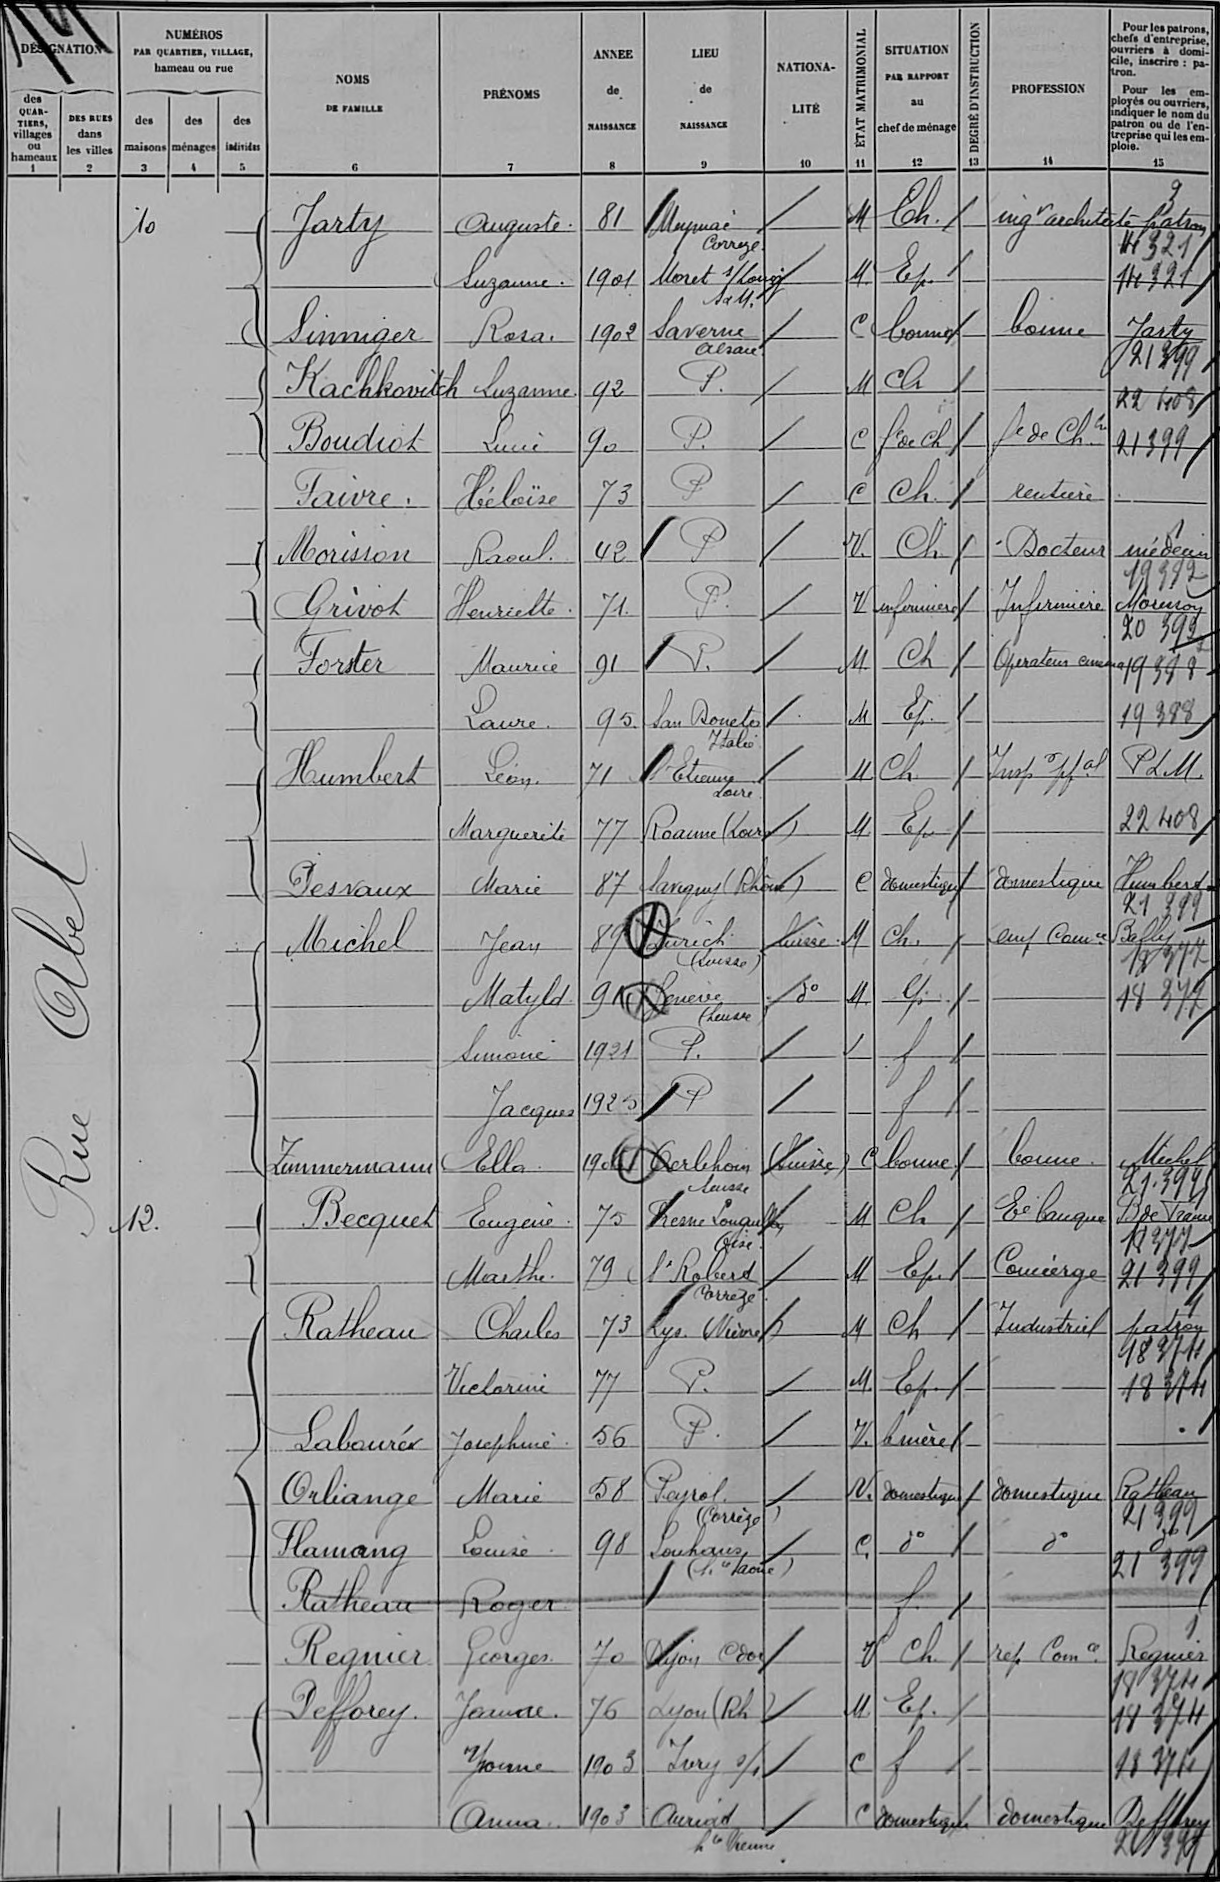Jarty/Auguste/81/meymac!correze/¤/M/Ch /¤/ingr architecte/patron!14321
¤/Suzanne/1901/Moret-Loing!S&M/¤/M/Ep /¤/¤/14321
Sinniger/Rosa/1902/Saverne!Alsace/¤/C/bonne/¤/bonne/Jarty!21399
Kachkovitch/Suzanne/92/P /¤/M/Ch/¤/¤/22408
Boudioh/Lucie/90/P /¤/C/Fe de Ch/¤/Fe de Ch/21399
Faivre/Héloïse/73/P/¤/C/Ch /¤/rentière/¤
Morisson/Raoul/42/P/¤/V /Ch/¤/Docteur/médecin!19382
Grivoh/Henriette/71/P /¤/V/Infirmière/¤/Infirmière/Moruroy !20392
Forster/Maurice/91/P /¤/M/Ch/¤/Opérateur cinema/19388
¤/Laure/95/San Donato!Italie/¤/M/Ep /¤/¤/19388
Humbert/Léon/71/St Etienne!Loire/¤/M/Ch/¤/Insp ppal PdM/¤
¤/Marguerite/77/Roanne (Loire)/¤/M/Ep/¤/¤/22408
Desnaux/Marie/87/Savigny (Rhône)/¤/E/domestique/¤/domestique/Humbert !21399
Michel/Jean/89/Zurich!(Suisse)/Suisse/M/Ch /¤/emp Comce/Bally!18377
¤/Matyld/91/Geneve!(Suisse)/d°/M/Ep /¤/¤/18372
¤/Simone/1921/P /¤/¤/f/¤/¤/¤
¤/Jacques/1925/P/¤/¤/f/¤/¤/¤
Zimmermann/Ella/1904/Oerlikon !Suisse/(Suisse)/C/bonne/¤/bonne/Michel!21399
Becquet/Eugene/75/Fresnes Longuillon !Oise/¤/M/Ch/¤/Eé banque/B de France!18377
¤/Marthe/79/St Robert !Corrèze/¤/M/Ep /¤/Concierge/21399
Ratheau/Charles/73/Lys (Nièvre)/¤/M/Ch/¤/Industriel/patron!18374
¤/Vieloruie/77/P /¤/M /Ep /¤/¤/18374
Labouré/Josephine/56/P /¤/V /b mère/¤/¤/¤
Orliange/Marie/58/Peyrol!(Corrèze)/¤/V /domestique/¤/domestique/Ratbeau!21399
Hamang/Louise/98/Louhans!(hte Saone)/¤/C/d°/¤/d°/d° !21399
Ratheau/Roger/¤/¤/¤/¤/f/¤/¤/¤
Regnier/Georges/70/Dijon Cdor/¤/V/Ch /¤/rep Comce/Regnier!18374
Defforey/Jeanne/76/Lyon (Rh)/¤/M/Ep /¤/¤/18194
¤/Yvonne/1903/Ivry/¤/C/f/¤/¤/18374
¤/Anna/1903/Auriat ! Hte Vienne/¤/C/domestique/¤/domestique/Defforey!21399
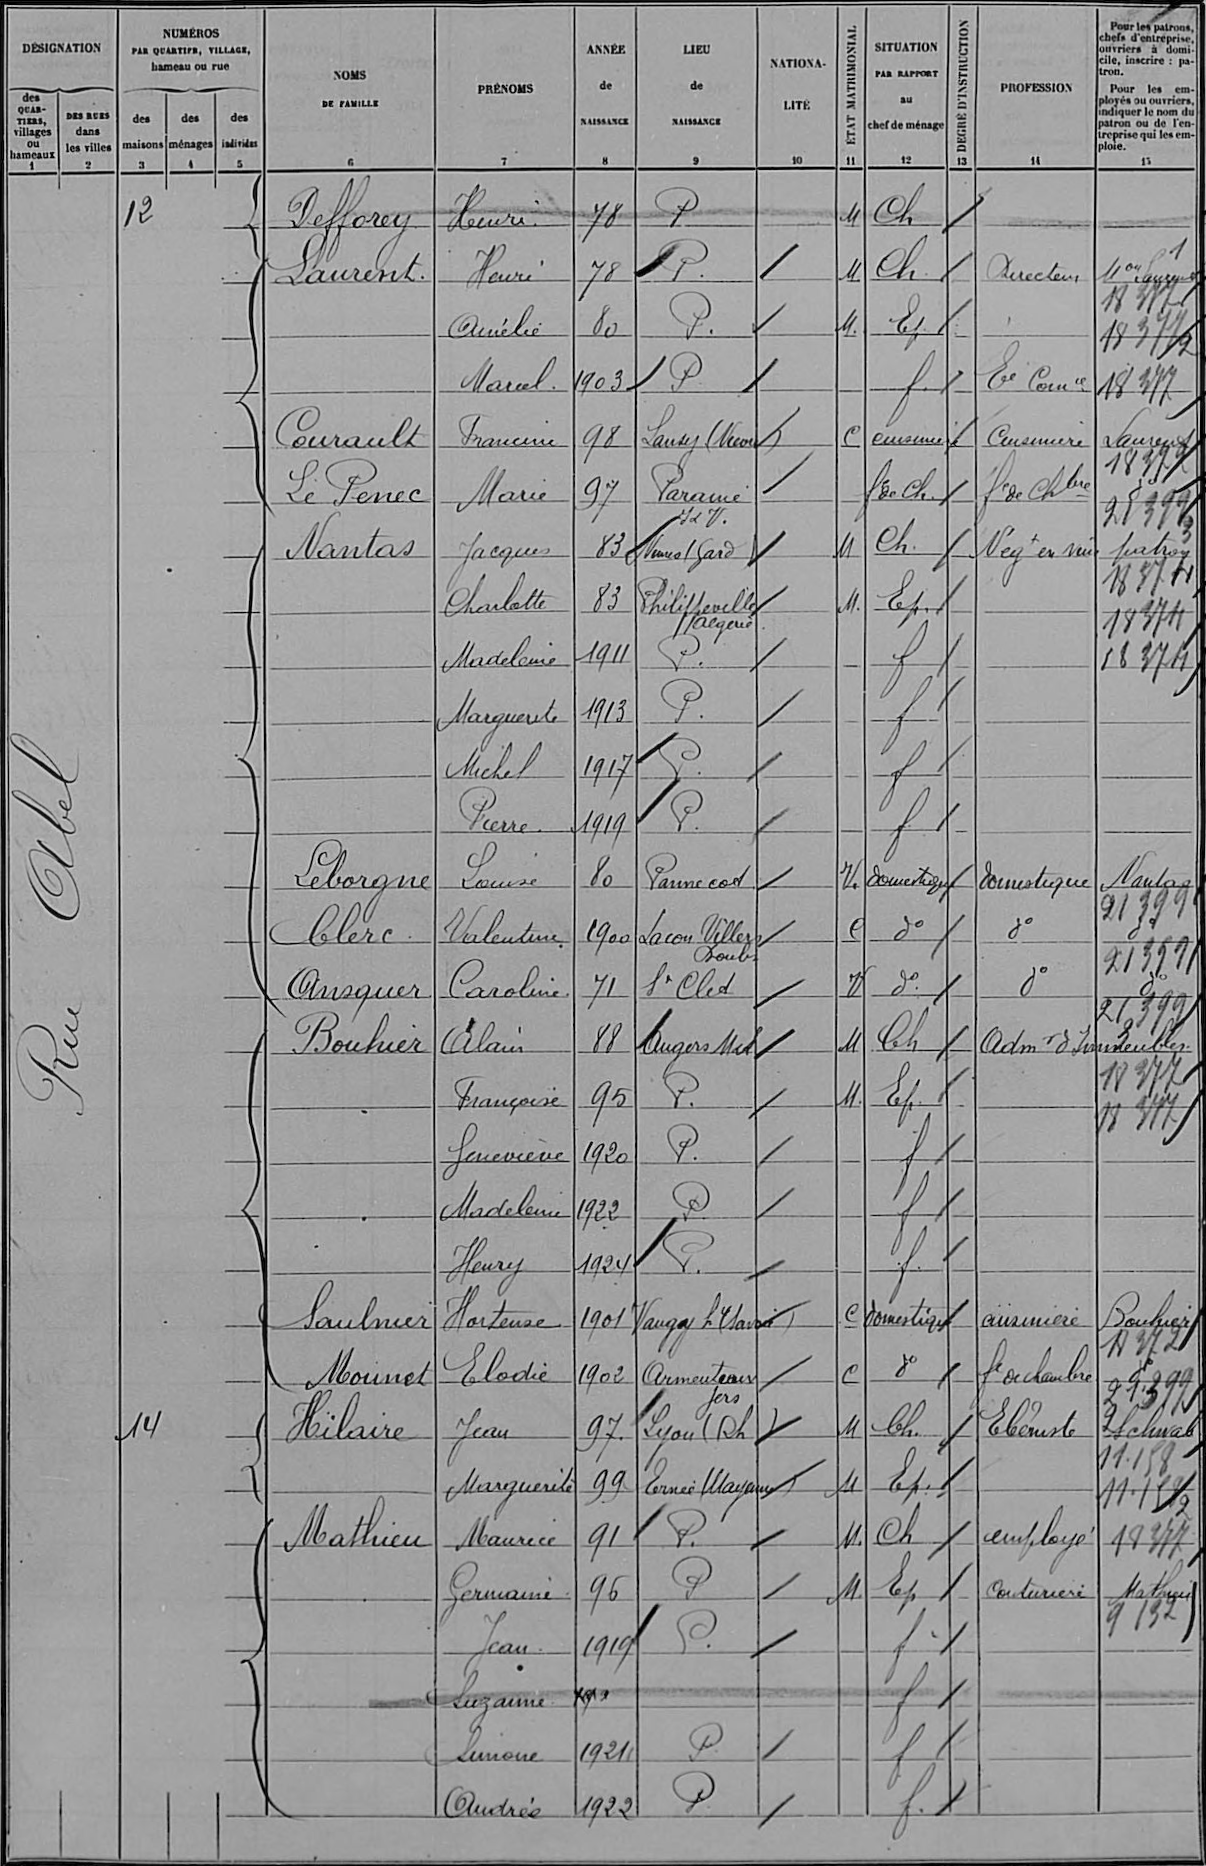Defforey/Henri/78/P/¤/M/Ch/¤/¤/¤
Laurent/Henri/78/P /¤/M/Ch /¤/Directeur/Mon Laurent!18377
¤/Amélie/80/P /¤/M /Ep/¤/¤/18377
¤/Marcel/1903/P/¤/¤/f /¤/Eé Comce/18377
Courault/Francine/98/Lausy (Nievres)/¤/C/cuisinière/¤/Cuisinière/Laurent!18372
Le Perrec/Marie/97/Paramé !I & V /¤/¤/f de Ch /¤/f de Chbre/d° !21399
Nantas/Jacques/83/Ners Gard/¤/M/Ch /¤/Negt en vins/patron!18874
¤/Charlotte/83/Philippeville!Algerie/¤/M /Ep /¤/¤/18374
¤/Madeleine/1911/P /¤/¤/f/¤/¤/18374
¤/Marguerite/1913/P /¤/¤/f/¤/¤/¤
¤/Michel/1917/P /¤/¤/f/¤/¤/¤
¤/Pierre/1919/P /¤/¤/f/¤/¤/¤
Leborgne/Louise/80/Paune cod/¤/V /domestique/¤/domestique/Nautae!21399
Clerc/Valentine/1900/Lacon Villers!Doubs/¤/E/d°/¤/d°/d° !21399
Ansquer/Caroline/71/St Clet/¤/V/d°/¤/d°/d° !21399
Bouhier/Alain/88/Angers M&L/¤/M/Ch/¤/Adm d Immeubles/!18377
¤/Françoise/95/P /¤/M /Ep /¤/¤/18377
¤/Genevieve/1920/P /¤/¤/f/¤/¤/¤
¤/Madeleine/1922/P /¤/¤/f/¤/¤/¤
¤/Henry/1924/P /¤/¤/f /¤/¤/¤
Saulnier/Hortense/1901/Vougy ht Savoie/¤/C/domestique/¤/cuisinière/Bouhier!18372
Mounet/Elodie/1902/Armentaux !Gers/¤/C/d°/¤/f de chambre/d° !21399
Hilaire/Jean/97/Lyon (Rh)/¤/M/Ch /¤/Ebeniste/2Schival!11158
¤/Marguerite/99/Ernée (Mayenne)/¤/M/Ep /¤/¤/11158
Mathieu/Maurice/91/P /¤/M /Ch/¤/employé/18377
¤/Germaine/96/P/¤/M/Ep/¤/couturière/Mathieu!9132
¤/Jean/1919/P/¤/¤/f/¤/¤/¤
¤/Suzanne/¤/¤/¤/¤/f/¤/¤/¤
¤/Simone/1921/P /¤/¤/f/¤/¤/¤
¤/Andrée/1922/P/¤/¤/f /¤/¤/¤